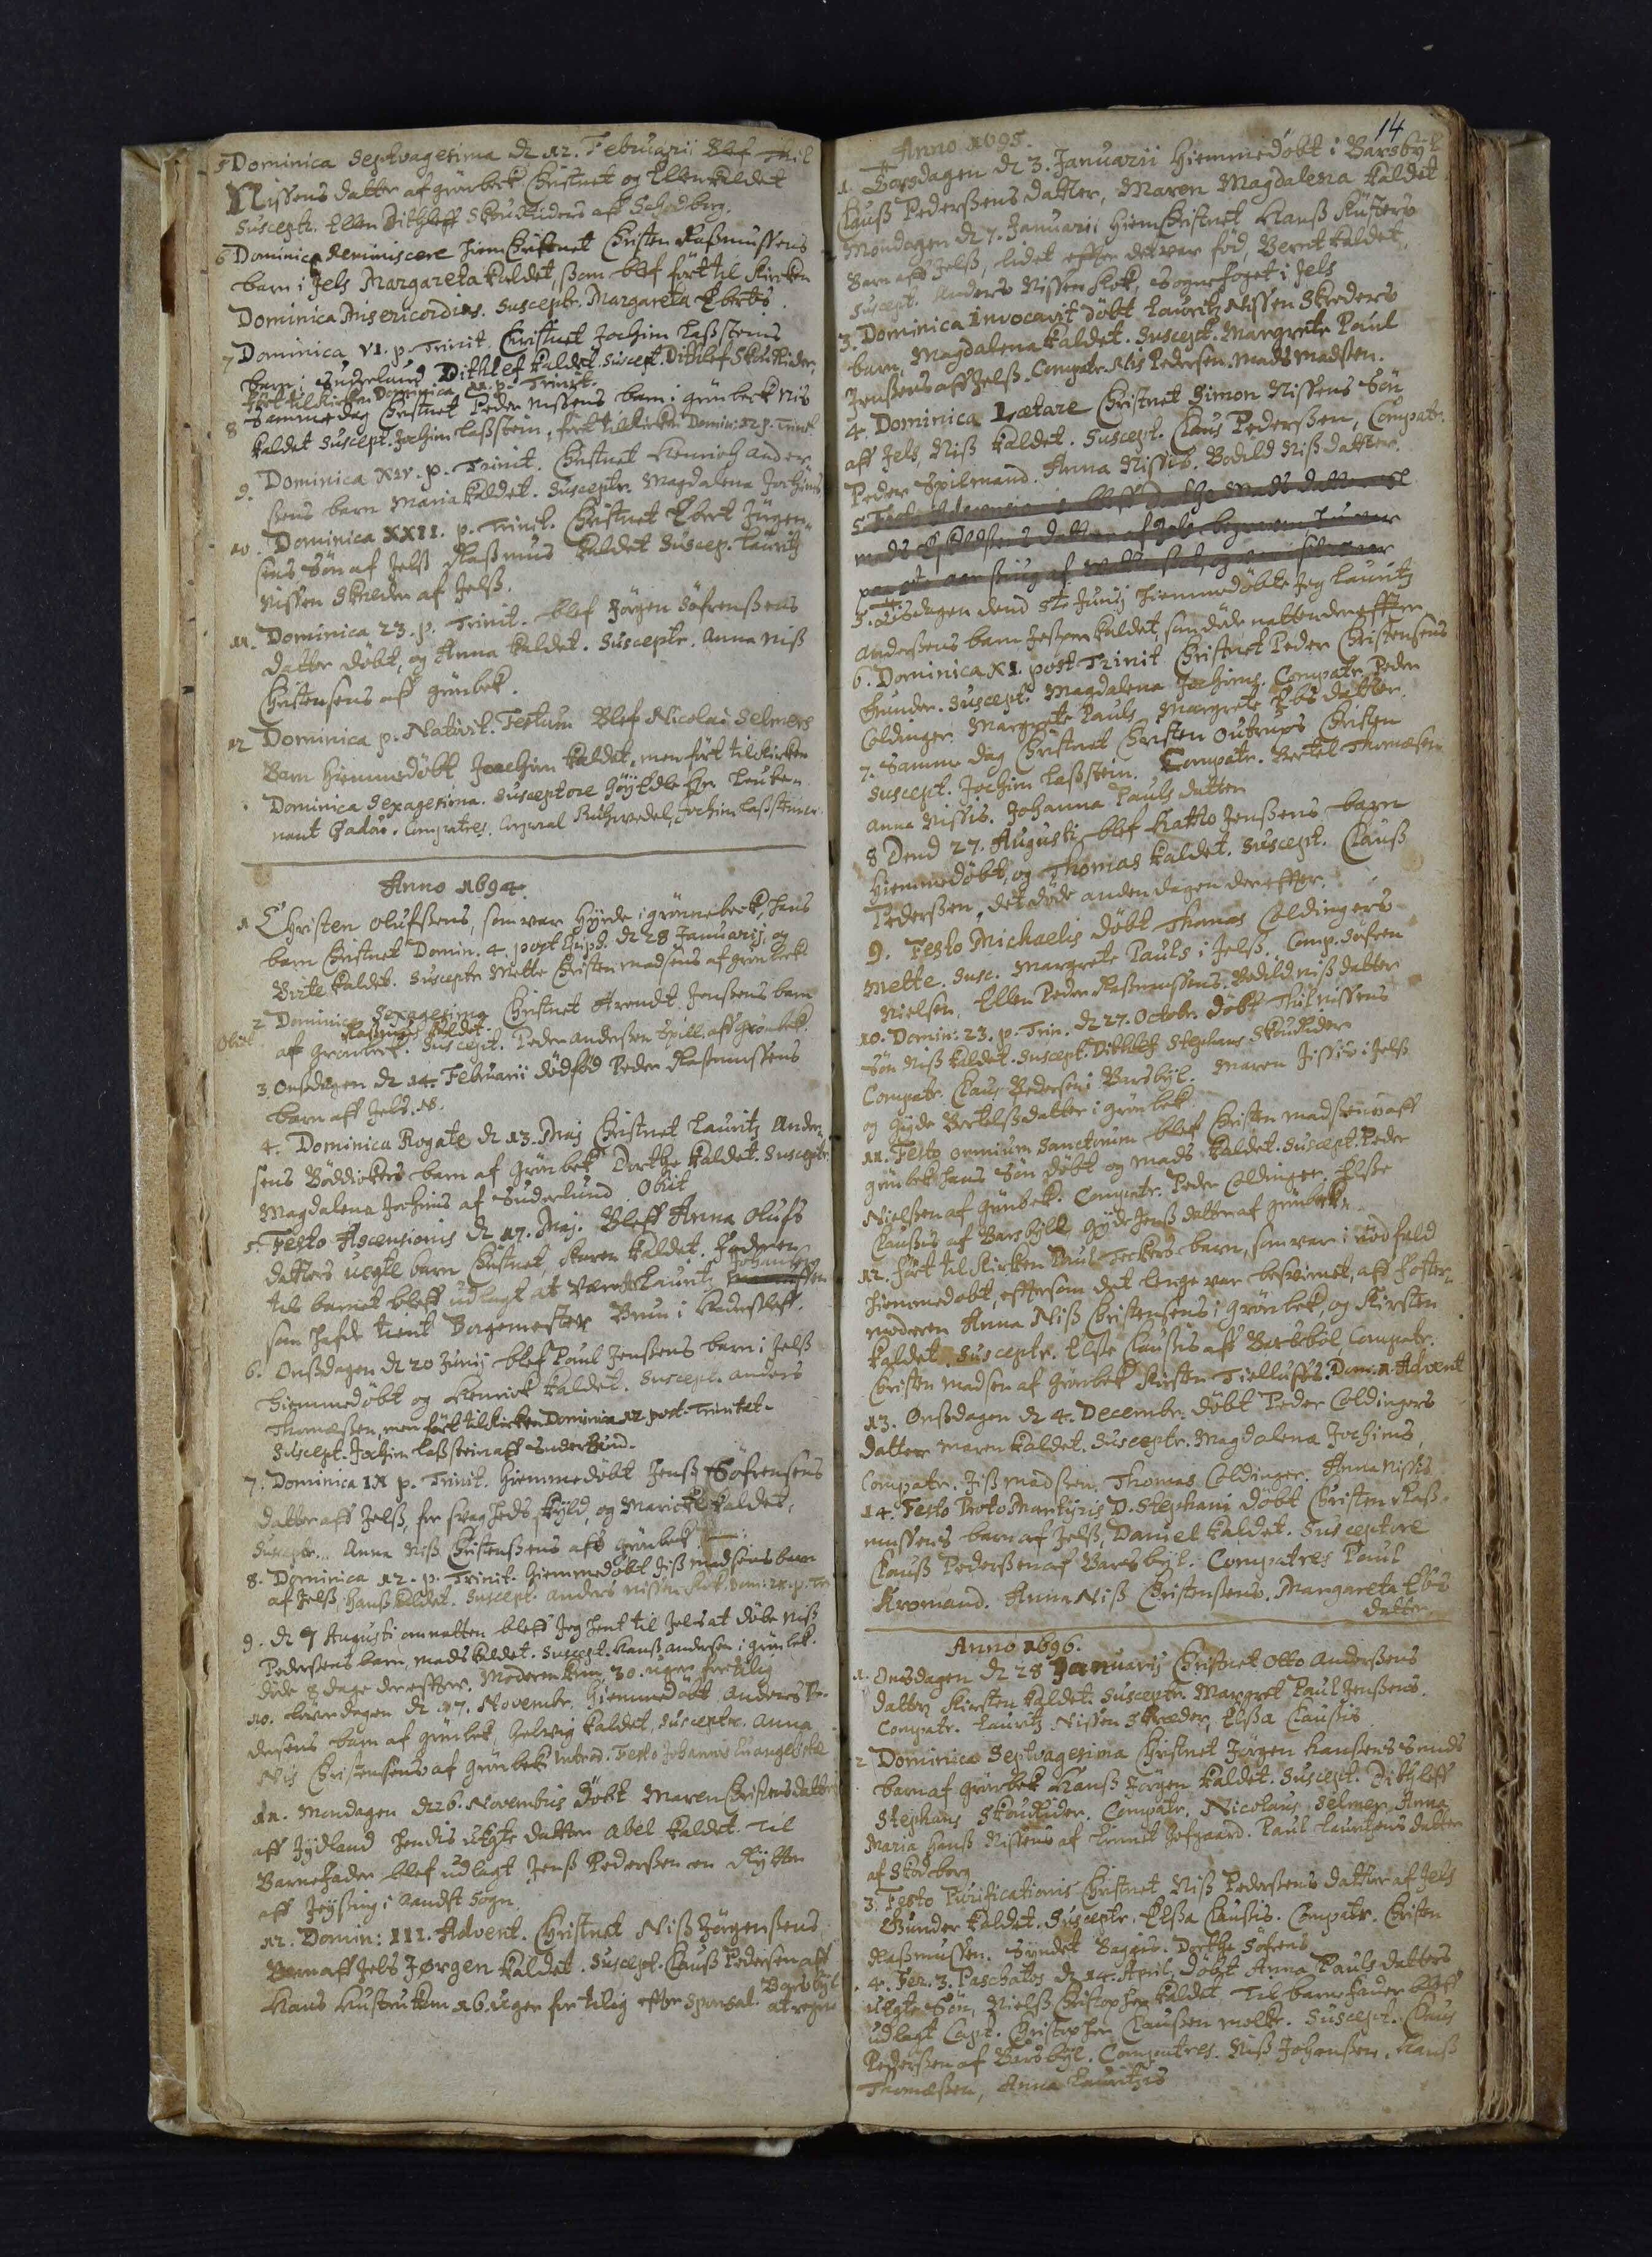5. Dominica Septvagesima d 12. Februarii Blef Thil
Nissens datter af grønbeck Christnet og Ellen kaldet
Susceptr. Ellen Dithleff SkouRiders aff Schodborg.
6. Dominica Reminiscere hiem Christnet Christen Rasmussens
barn i Jels Margareta kaldet, som blef ført til Kirke
Dominica Misericordias. Susceptr. Margareta Ebertis.
7. Dominica VI. p. Trinit. Christnet Jochim Lassteins
barn i Suderlund Dithlef kaldet. Suscept. Dithlef Skou Rider,
ført til kirken Dominica 11. p. Trinit.
8. Samme dag Christnet Peder Nissens barn i grønbeck Nis
kaldet. Suscept. Jochim Lasstein, ført til Kirke Domin. 12. p. Trinit.
9. Dominica XIV. p. Trinit. Christnet Henrich Ander¬
sens barn Maria kaldet. Susceptr. Magdalene Jochims
10. Dominica XXII. p. Trinit. Christnet Ebert Jurgen¬
sens Søn af Jels Rasmus kaldet. Suscep. Lauritz
Nissen Skreder af Jels.
11. Dominica 23. p. Trinit. blef Jørgen Søfrensens
datter døbt, og Anna kaldet. Susceptr. Anna Niss
Christensens aff grønbeck.
12. Dominica p. Nativ ##. Festum. Blef Nicolai Selmers
Barn Hiemmedøbt Joachim kaldet, men ført til Kirken
Dominica Sexagesima. Susceptore Gøy## Hr. Leuten
nant Gadov. Comjutres## Corporal Ruhnadel## Jochim Lastein.
Anno 1694.
1 Christen Olufsens, som var hyrde i grønnebeck, hans
barn Christnet Domin. 4. post Epiph. d 28 Januarij, og
Birte kaldet. Susceptr. Mette Christen madsens af grønbeck.
2 Dominica Sexagesima Christnet Arendt Jensens barn
Rasmus kaldet.
obiit
aff Grønbeck. Suscept. Peder andersen Spilm. aff grønbek.
3. Onsdagen d 14. Februarii dødfød Peder Rasmussens
barn aff Jels. NB.
4. Dominica Rogate d 13. Maj Christnet Lauritz Ander¬
sens Bøddickers barn aff Grønbek Dorthe kaldet. Suscept.
Magdalena Jochims af Sudurlund. Obiit.
5. Festo Ascensionis 17. Maj. Bleff Anna Olufs
datters uegte barn Christnet, Karen kaldet. Faderen
til barnet bleff udlagt at være Lauritz
Johansen
som hafde tient Borgermester Brun i Hadersleff.
6. Onsdagen d 20 Junij blef Poul Jensens barn i Jels
hiemmedøbt og Henrick kaldet. Suscept. Anders
Thomæsen, men ført til Kirken Dominica 12 post Trinitat.
Suscept. Joachim Lasstein aff Suderlund.
7. Dominica IX p. Trinit. hiemmedøbt Jens Søfrensens
datter aff Jels, for svagheds skyld, og Maricke kaldet,
Suscept. Anna Niss Christensens aff Grønbeck.
8. Dominica 12 p. Trinit. hiemmedøbt Jess Madsens barn
af Jels Hans kaldet. Suscept. Anders Nissen Kock. Dom: ## p. Tr
9. d 7 Augusti om natten bleff jeg hent til Jels at døbe Niss
Pedersens barn Mads kaldet. Suscept. Hans andersen i grønbeck.
døde 8 dage dereffter. Moderen kom 30. uger for tilig
10. Løverdagen d 17. November hiemmedøbt Anders Pe¬
dersens barn af grønbeck, Helwig kaldet. Susceptr. Anna
Nis Christensens af grønbeck. Introd. Festo Johannis Evangeliske
11. Mandagen d 26 Novembris døbt Maren Christens datter
aff Jydland hendis uEgte datter Abel kaldet til
Barnefader blef udlagt Jenss Pedersen en Rytter
af Jeysing i Aandst Sogn.
12. Domin: III. Advent. Christnet Niss Jørgensens
Barn aff Jels Jørgen kaldet. Suscept. Claus Pedersen aff
Barsbyl
Hans Hustru kom 16. uger for tilig effter Sjensal## at regne
14
Anno 1695.
1. Torsdagen d 3. Januarii Hiemmedøbt i Barsbyl
Claus Pedersens Datter, Maren Magdalena kaldet.
Mandagen d 7. Januarii Hiem Christnet Hans Küsters
Barn aff Jels, lidet effter det var fød, Bernt kaldet.
Suscept. Anders Nissen Kok, Sognefoget i Jels.
3. Dominica Invocavit døbt Lauritz Nissen Skreders
barn, Magdalena kaldet. Suscept. Margrete Poul
Iversens aff Jels. Compatr. Nis Pedersen. Mads Madsen.
4. Dominica Lætare Christnet Simon Nissens Søn
aff Jels Niss kaldet. Suscept. Claus Pedersen, Compatr.
Peder Spilmand. Anna Nissis. Bodild Nissdatter.
5. Festo Adscensio I bleff Dorthe Madsdatter sl
Mads Eskildsens 2 datter af Jels begraven, hun var
paa to aar siug af vattersot, og var i sit 25 aar.
5. Tisdagen dend 5te Junij hiemmedøbte jeg Lauritz
Andersens barn Jesper kaldet, som døde natten dereffter
6. Dominica XI post Trinit Christnet Peder Christensens
Gunder. Suscept. Magdalena Jochims. Compatr. Peder
Coldinger. Margrete Pauls Margrete Ebsdatter.
7. Samme dag Christnet Christen Outrups Christen
Suscept. Jochim Lasstein. Compatr. Bertel Thomæsen
Anna Nissis. Johanna Pauls datter
8 Dend 27. Augusti bleff Hatto Jensens barn
Hiemmedøbt og Thomas kaldet. Suscept. Claus
Pedersen. Det døde anden dagen dereffter.
9. Festo Michaelis døbt Thomas Coldingers
Mette. Susc. Margrete Pauls i Jels. Comp. Søfren
Nielsen. Ellen Peder Rasmussens. Bodild Niss datter.
10. Domin. 23 p. Trin. d 27. Octobr. Døbt Thil Nissens
Søn Niss kaldet. Suscept. Dithlef Stephans SkouRider
Compatr. Claus Pedersen i Barsbyl. Maren Jissis## i Jels
og Gyde Bertelsdatter i Grønbeck.
11. Festo Ommium Sanctum bleff Christen Madsens aff
Grønbeck hans Søn døbt og Mads kaldet. Suscept. Peder
Nielsen af Grønbek. Compatr. Peder Coldinger. Elsse
Clauses af Barsbyll, Gyde Jenss datter af Grønbek.
12. Ført til Kirken Paul Teckers barn, som var i nødfald
hiemmedøbt, eftersom det lenge var besvimet, aff Foster¬
moderen Anna Niss Christensens i Grønbeck, og Kirsten
kaldet. Susceptr. Else Clausis aff Barsbøl. Compatr.
Christen Madsen af Grønbeck. Kirsten Tielluses. Dom. 1. Advent.
13. Onsdagen d 4. Decembr. døbt Peder Coldingers
datter Maren kaldet. Susceptr. Magdalena Jochims,
Compatr. Jiss Madsen. Thomas Coldinger. Anna Nissis.
14. Festo Proto Martyris## D. Stephani døbt Christen Ras¬
mussens barn af Jels, Daniel kaldet. Susceptore
Claus Pedersen af Barsbyl. Compatres Poul
Kromand. Anna Niss Christensens. Margareta Ebs
datter.
Anno 1696.
1. Onsdagen d 28 Januarij Christnet Otto Andersens
datter Kiersten kaldet. Susceptr. Margret Paul Jensens.
Compatr. Lauritz Nielsen Skreder, Elssa Clausis
2. Dominica Septvagesima Christnet Jørgen Hansens Smids
barn af Grønbek Hans Jørgen kaldet. Suscept. Dithleff
Stephans SkouRider. Compatr. Nicolaus Selmer Anna
Maria Hans Nielsens af Linnet Hofgaard. Paul Lauritzens datter
af Skodborg.
3. Festo Purificationis Christnet Niss Pedersens datter af Jels
Gunder kaldet. Susceptr. Elssa Clausis. Compatr. Christen
Rasmussen. Sÿndet Bagis. Dorthe Søfrens
4. Fer. 3. Paschatos d 14. April Døbt Anna Pauls datters
uegte Søn, Niels Christopher kaldet. Til barnefader bleff
udlagt Capt. Christopher Clausen Molke. Suscept. Claus
Pedersen af Barsbyl. Compatres. Niss Johansen. Hans
Thomæsen, Anna Lauritzes